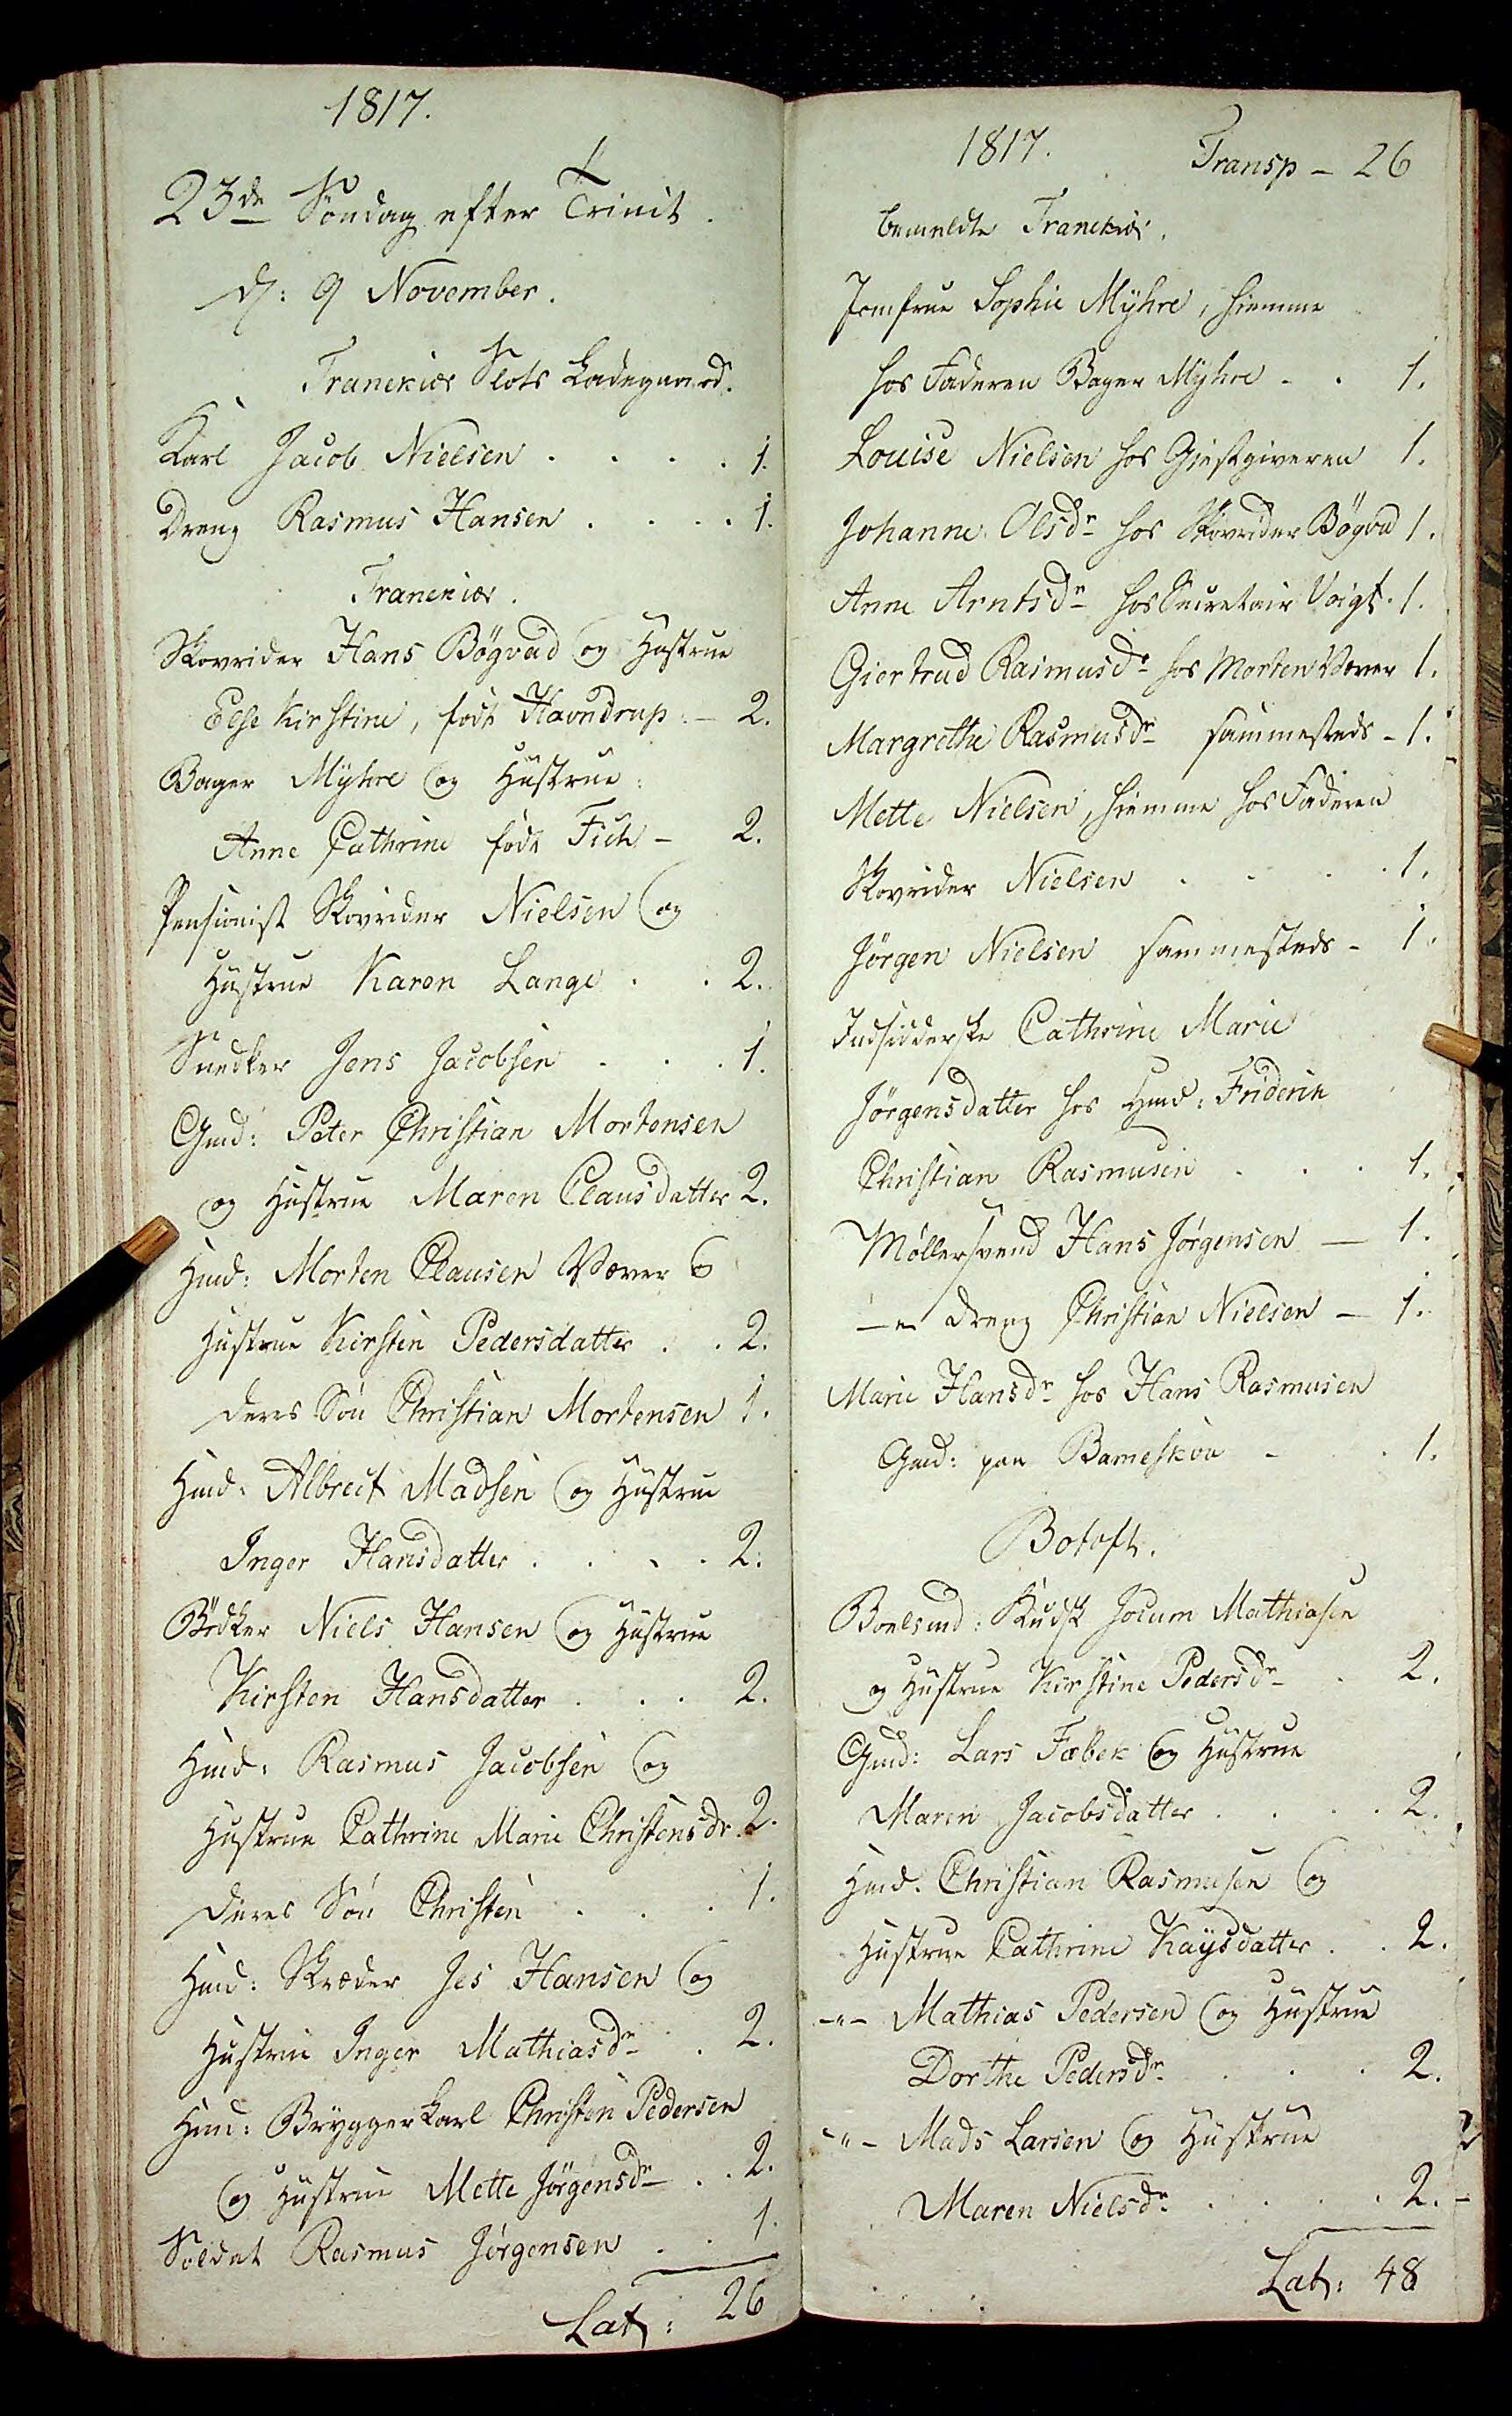1817.
23de Søndag efter Trinit.
d: 9 November.
Tranekiær Slots Ladegaard.
Karl Jacob Nielsen . . . .1.
Dreng Rasmus Hansen . . . . 1
Tranekiær.
Skovrider Hans Bøgvad og Hustrue
Else Kirstine, født Havndrup . - 2.
Bager Myhre og Hustrue
Anne Cathrine født Fich - 2.
Pensionist Skovrider Nielsen og
Hustrue Karen Lange . .  2.
Snedker Jens Jacobsen . . . 1.
Gmd: Peder Christian Mortensen
og Hustrue Maren Clausdatter 2.
Hmd: Morten Clausen Væver og
Hustrue Kirsten Pedersdatter . . 2.
deres Søn Christian Mortensen 1.
Hmd: Albrect Madsen og Hustrue
Inger Hansdatter . . . . 2.
Bødker Niels Hansen og Hustrue
Kirsten Hansdatter . . . 2.
Hmd: Rasmus Jacobsen og
Hustrue Cathrine Marie Christensdr . 2.
deres Søn Christen . . . 1
Hmd: Skræder Jes Hansen og
Hustrue Inger Mathiasdr . 2
Hmd: Bryggerkarl Christen Pedersen
og Hustrue Mette Jørgensdr . . 2.
Soldat Rasmus Jørgensen . . 1.
Lat: 26
1817.
Transp - 26
bemeldte Tranekiær.
Jomfrue Sophie Myhre, hiemme
hos Faderen Bager Myhre . .1.
Louise Nielsen hos Giestgiveren 1.
Johanne Olsdr hos Skovrider Bøvad 1.
Anne Arntsdr hos Secretair Voigt . 1.
Giertrud Rasmusdr hos Morten Væver 1.
Margrethe Rasmusdr sammesteds - 1.
Mette Nielsen, hiemme hos Faderen
Skovrider Nielsen . . . . 1.
Jørgen Nielsen sammesteds - 1.
Indsidderske Cathrine Marie
Jørgensdatter hos Hmd: Friderik
Christian Rasmusen . . . 1.
Møllersvend Hans Jørgensen - 1.
-"- dreng Christian Nielsen - 1.
Marie Hansdr hos Hans Rasmusen
Gmd: paa Bameskov - . . 1.
Botoft.
Boelsmd: Kudsk Jocum Mathiasen
og Hustrue Kirstine Pedersdr . 2.
Gmd: Lars Fæbek og Hustrue
Maren Jacobsdatter . . . . 2.
Hmd. Christian Rasmusen og
Hustrue Cathrine Kaysdatter . . 2.
-"- Mathias Pedersen og Hustrue
Dorthe Pedersdr . . . 2.
-"- Mads Larsen og Hustrue
Maren Nielsdr . . . . 2.
Lat: 48
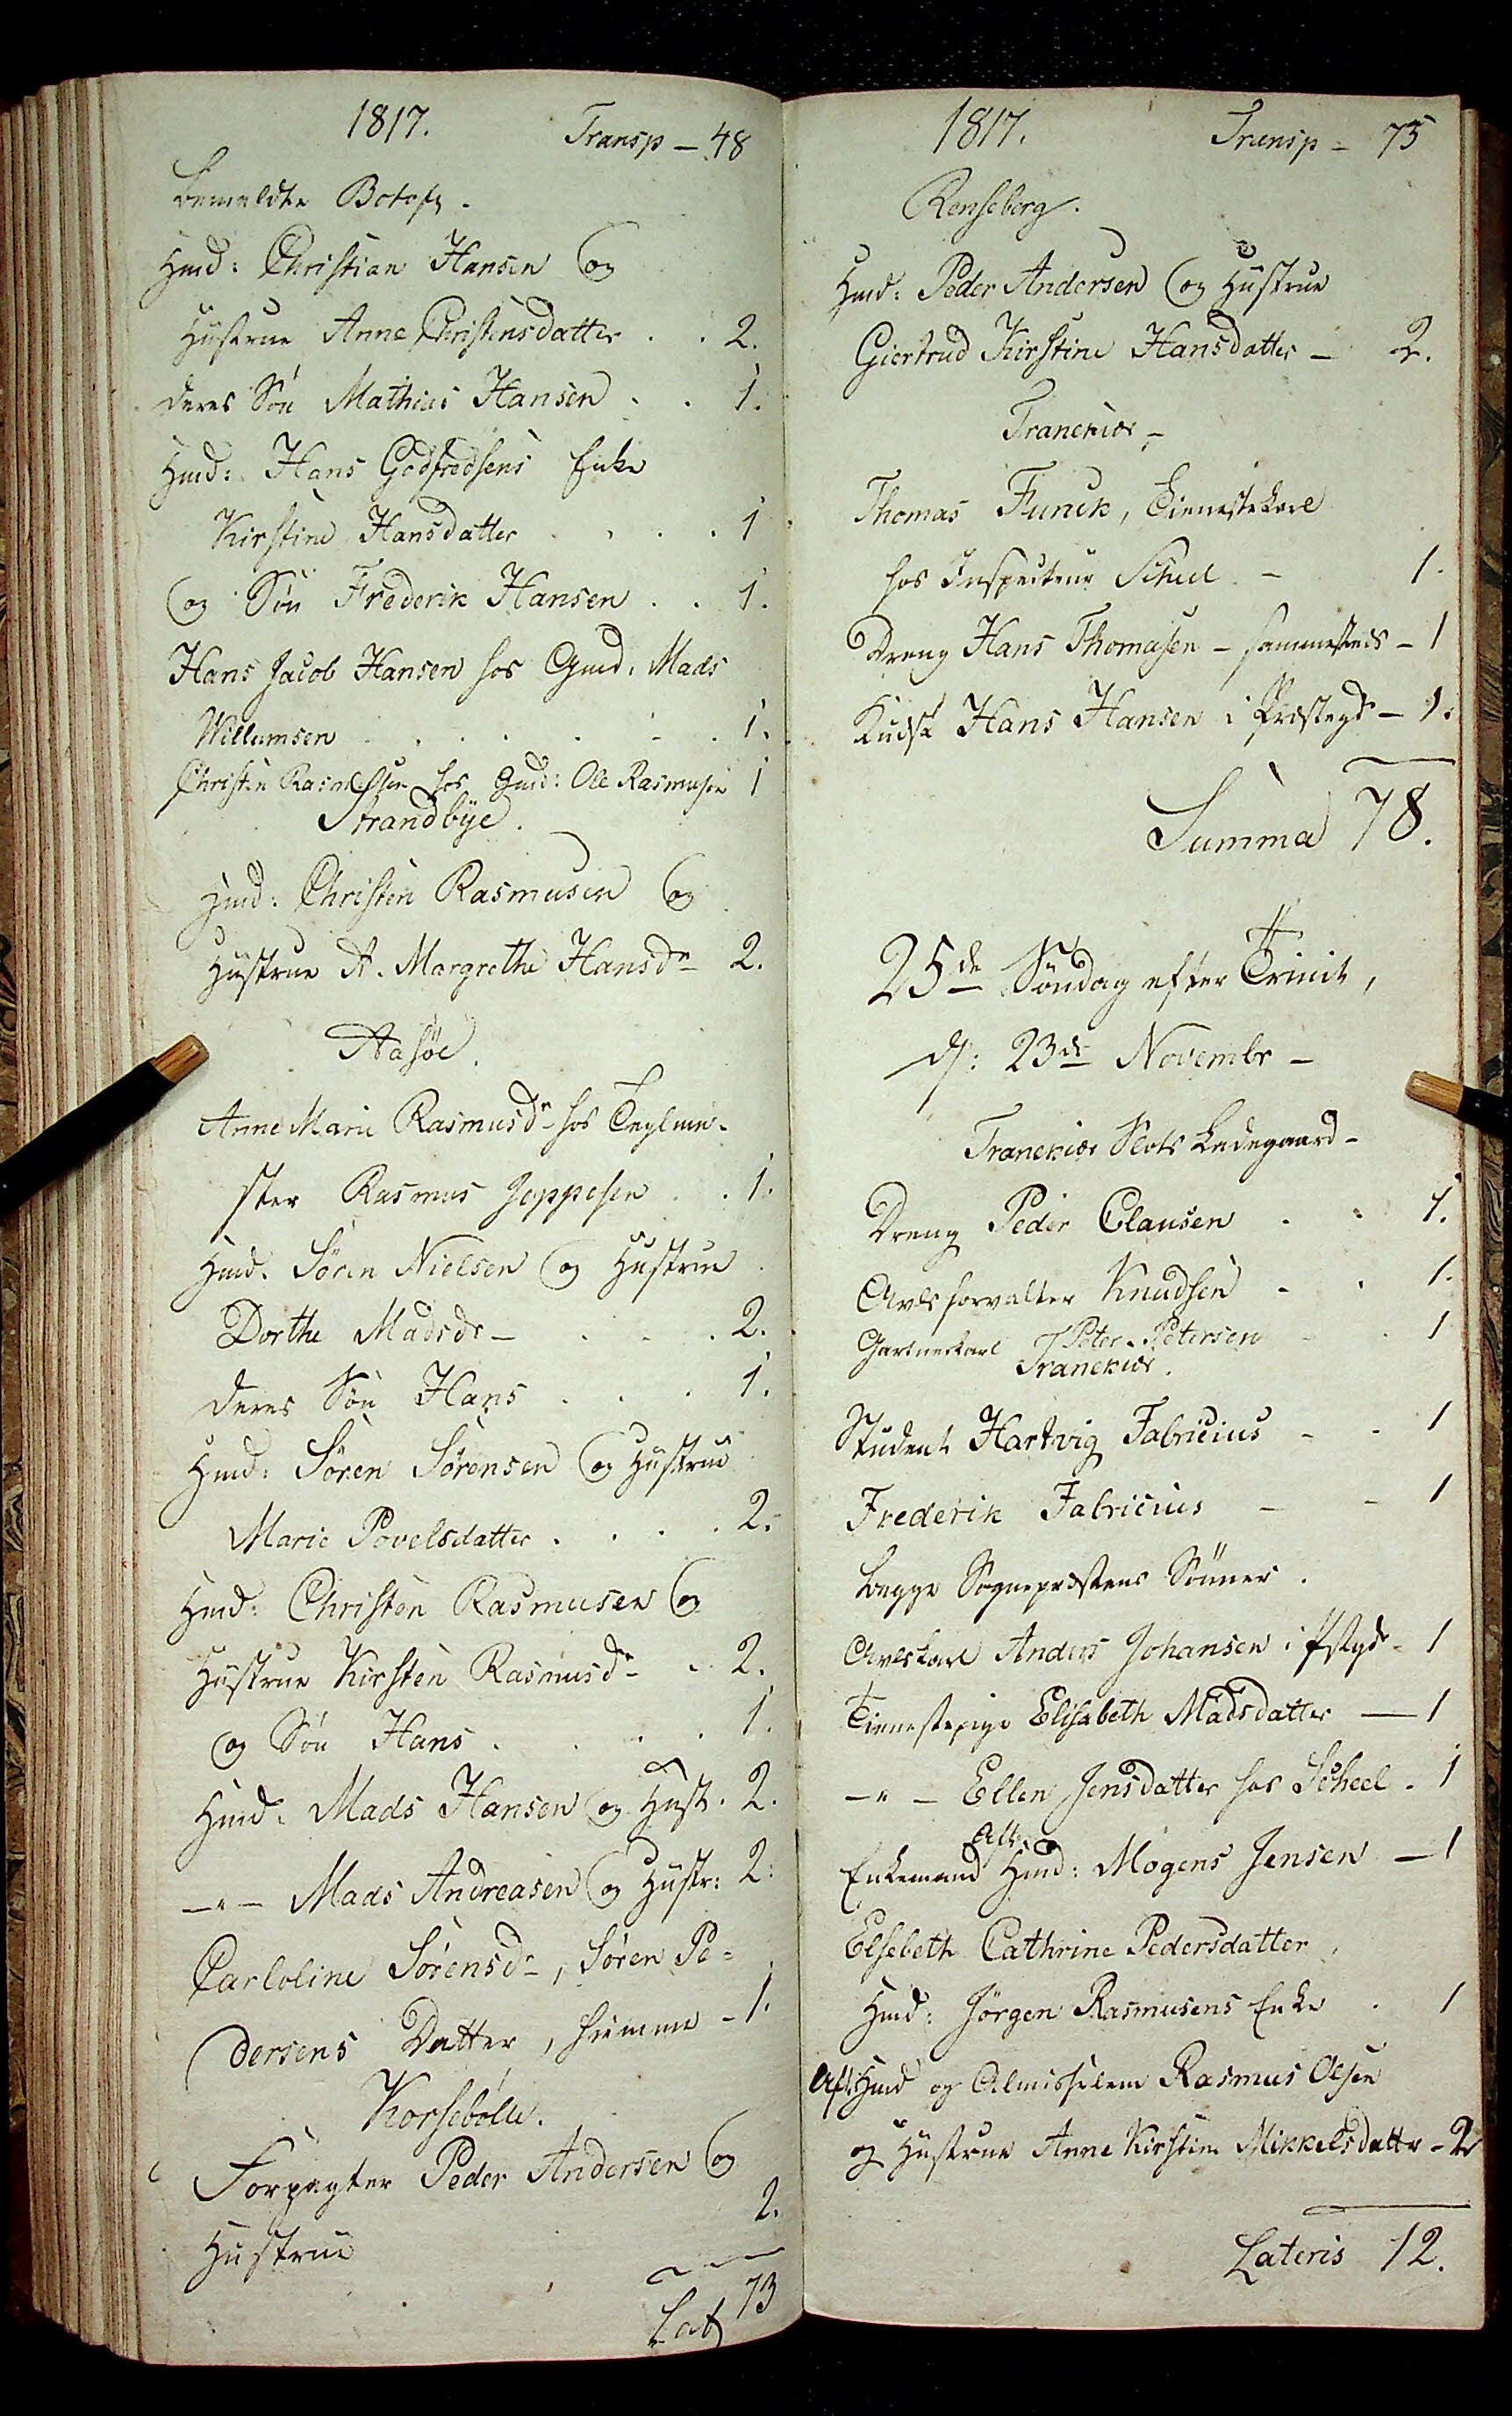1817.
Transp - 48
bemeldte Botoft.
Hmd: Christian Hansen og
Hustrue Anne Christensdatter . . 2.
deres Søn Mathias Hansen . . 1.
Hmd: Hans Godfredsens Enke
Kirstine Hansdatter . . . . 1
og Søn Friderik Hansen . . 1.
Hans Jacob Hansen hos Gmd: Mads
Willumsen . . . . . . . 1.
Christen Rasmusen hos Gmd: Ole Rasmusen 1
Strandbye.
Hmd: Christen Rasmusen og
Hustrue A. Margrethe Hansdr 2.
Aasøe.
Anne Marie Rasmusdr hos Teglme¬
ster Rasmus Jeppesen . . 1.
Hmd: Søren Nielsen og Hustrue
Dorthe Madsdr - . . .2.
deres Søn Hans . . . 1.
Hed: Søren Sørensøn og Hustrue
Marie Povelsdatter . . . . 2.
Hmd: Christen Rasmusen og
Hustrue Kirsten Rasmusdr . 2.
og Søn Hans . . . . 1.
Hmd: Mads Hansen og Hust . 2.
-"- Mads Andreasen og Hustr: 2:
Carloline Sørensdr, Søren Pe¬
dersens Datter, hiemme - 1.
Korsebølle.
Forpagter Peder Andersen og
Hustrue . 2.
Lat 73
1817.
Transp - 75
Renseberg.
Hmd: Peder Andersen og Hustrue
Giertrud Kirstine Hansdatter - 2.
Tranekiær, -
Thomas Funck, Tienestekarl
hos Inspecteur Scheel - 1.
Dreng Hans Thomasen - sammesteds - 1
Kudsk Hans Hansen i Præstegd - 1.
Summa 78.
25de Søndag efter Trinit,
d: 23de Novembr-
Tranekiær Slots Ladegaard-
Dreng Peder Clausen . . 1.
Avlsforvalter Knudsen . . 1.
Gartnerkarl Peter Petersen . . 1
TYranekiær.
Student Hartvig Fabricius - . 1
Frederik Fabricius - - 1
begge Sognepræstens Sønner
Avlskarl Anders Johansen i Pstgd - 1
Tienestepige Elisabeth Madsdatter - 1
-"- Ellen Jensdatter hos Scheel . 1
Aft
Enkemand Hmd: Mogens Jensen - 1
Elsebeth Cathrine Pedersdatter
Hmd: Jørgen Rasmusens Enke . 1
Aft. Hmd og Almisselen Rasmus Olsen
og Hustrue Anne Kirstine Mikkelsdatter - 2
Lateris 12.